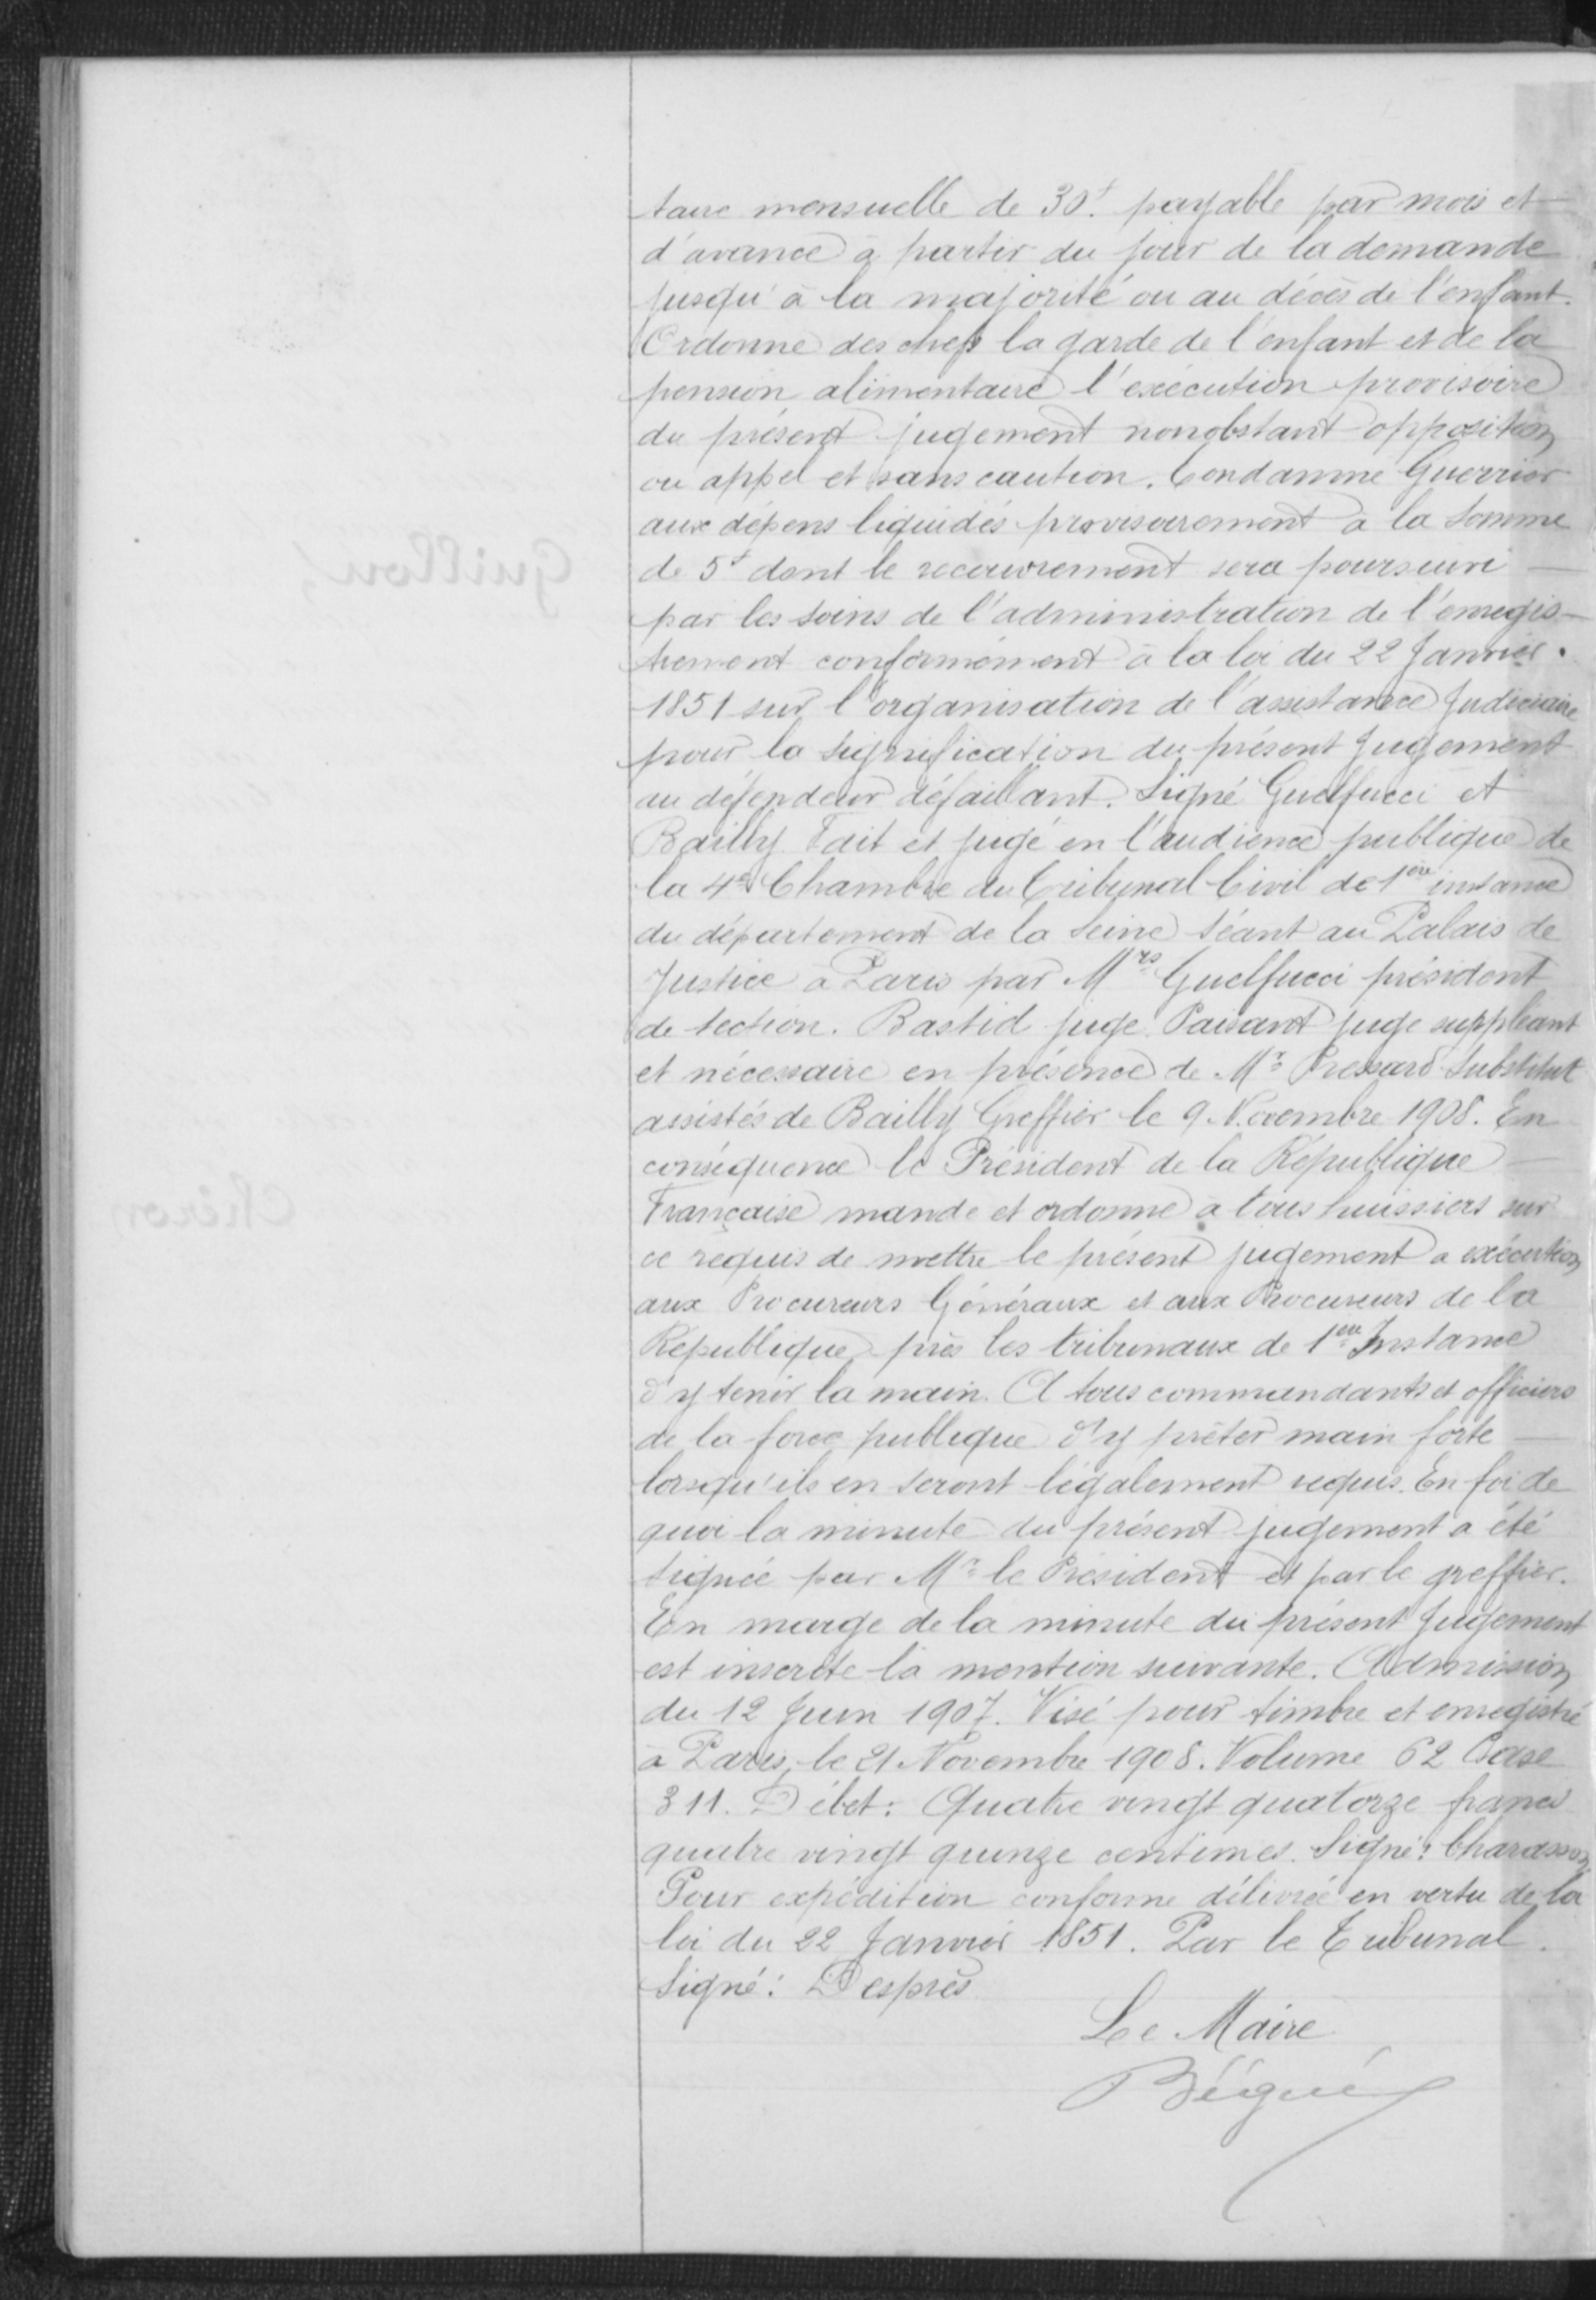taire mensuelle de 30t payable par mois et
d'avant à partir du jour de la demande
jusqu'à la majorité ou au décès de l'enfant.
Ordonne des chefs la garde de l'enfant et de la
pension alimentaire l'exécution provisoire
du présent jugement nonobstant opposition,
ou appel et sans caution. condamne Guerrier
aux dépens liquidés provisoirement à la Somme
de 5f dont le recouvrement sera poursuivi
par les soins de l'administration de l'enregis-
trement conformément à la loi du 22 Janvier
1851 sur l'organisation de l'assistance Judiciaire
pour la signification du présent Jugement
au defendeur défaillant. Signé Guelfucci et
Bailly. Fait et jugé en l'audience publique de
la 4° Chambre du Tribunal Civil de 1ere instance
du département de la Seine Séant au Palais de
Justice à Paris par Mre Guelfucci président
de section. Bastid Juge Pauvant juge suppléant
et nécessaire en présence de Me Preysard Substituant
assistés de Bailly Greffier le 9 Novembre 1909. En
conséquence el Président de la République
Française mande et ordonne à tous huissiers sur
ce requis de mettre le présent jugement a exécution
aux Procureur Généraux et aux Procureurs de la
République près les tribunaux de 1ere Instance
d'y tenir la main. Et tous commandants et officier
de la force publique s'y prêter main forte
lorsqu'ils en seront légalement requis. En foi de
quoi la minorité du présent jugement a été
signée par Mr le Président et par le greffier
En marge de la minute du présent Jugement
est inserte la mention suivante. Admission
du 12 Juin 1907. Visé pour timbre et enregistré
à Paris le 21 Novembre 1908. Volume 62 Cas
311. Débat: Quatre vingt quatorze franc
quatre vingt quinze centimes Signé: Charrassoz
Pour expédition conforme délivré en vertu de la
loi du 22 Janvier 1851. Par le Tribunal
Signé: Despres.
Le Maire
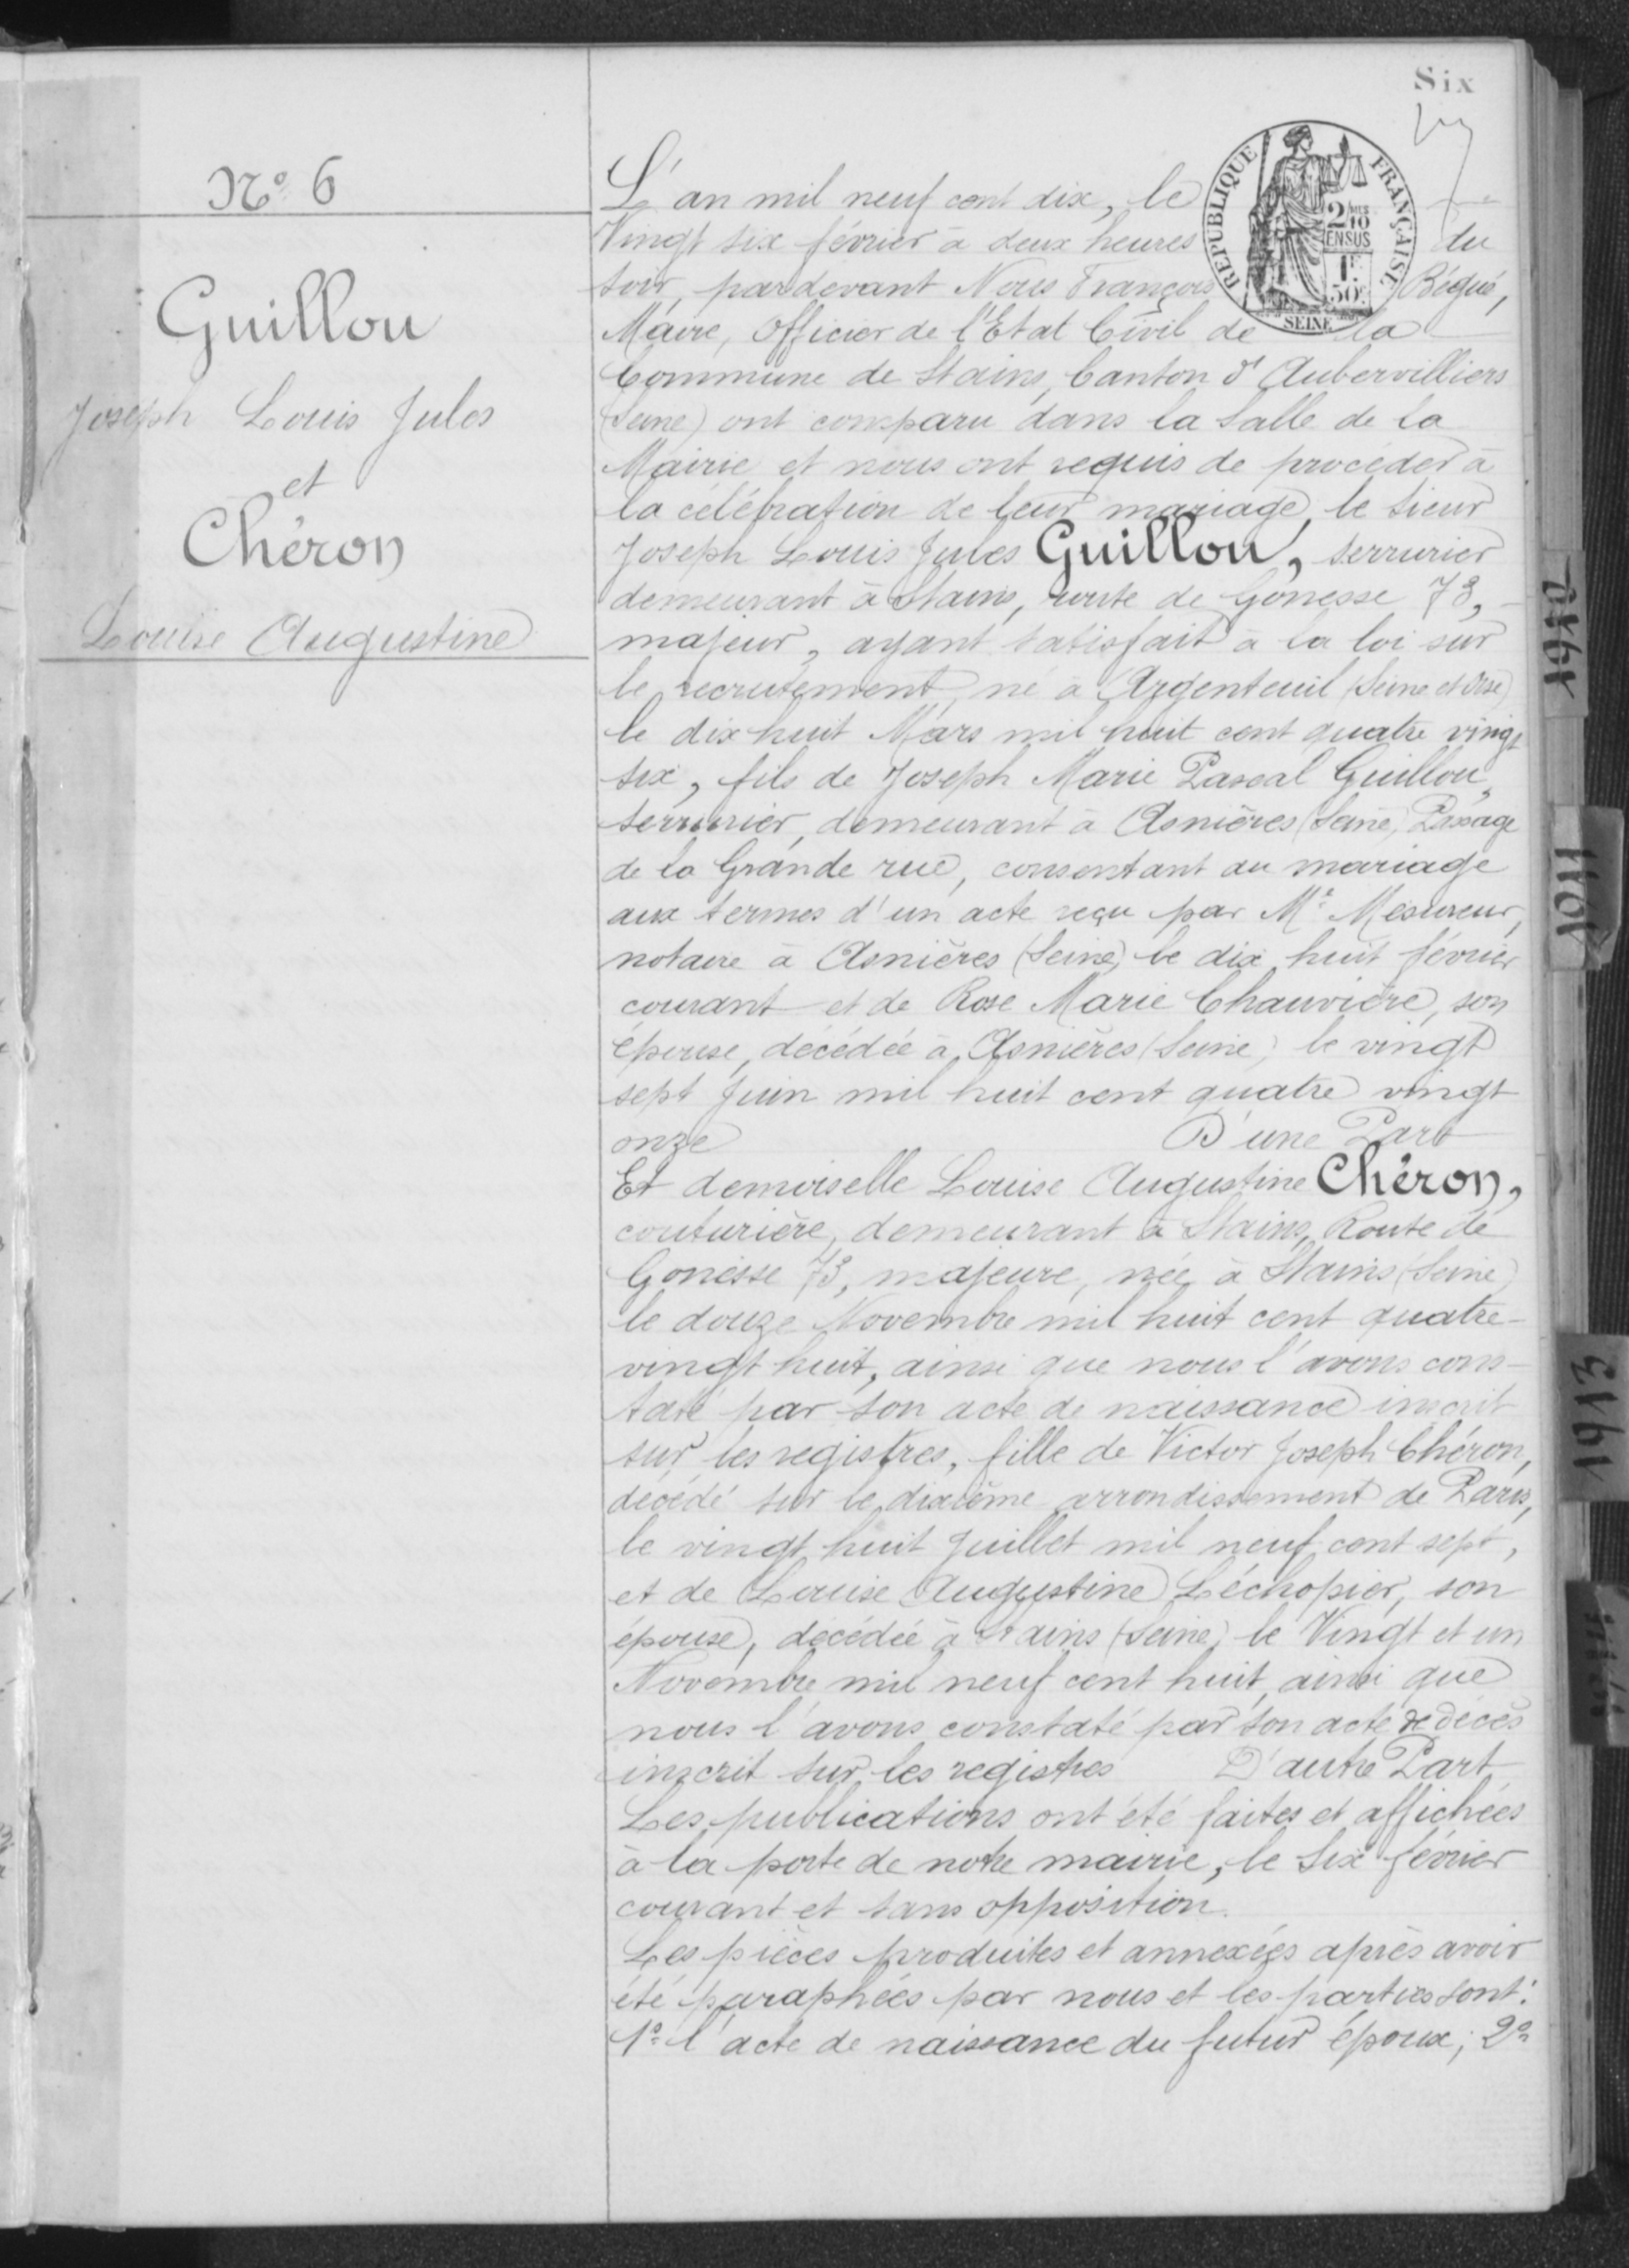N°6
Guillou
Joseph Louis Jules
et
Chéron
Louise Augustine
L'an mil neuf cent six, le
Vingt six février à deux heures du
soir, par devant Nous François Bégus,
Maire, Officier de l'Etat Civil de la
Commune de Stains, Canton d'Aubervillies
(Seine) ont comparu dans la Salle de la
mairie et nous ont requis de procéder à
la célébration de leur mariage le Sieur
Joseph Louis Jules Guillou, serrurier
demeurant à Stains, route de Gonesse, 73,
majeur, ayant satisfait à la loi sur
le recrutement, né à Argenteuil (Seine et Oise)
le dix huit Mars mil huit cent quatre vingt
six, fils de Joseph Marie Pasoal Guillou,
serrurier, demeurnt à Asnières (Seine) Laxage
de la Grande rue, consentant au mariage
aux termes d'un acte reçu par Me Mesureur,
Notaire à Asnières (Seine) le dix huit février
courant et de Rose Marie Chauvrière, son
épouse, décédée à Asnières (Seine) le vingt
sept juin mil huit cent quatre vingt
onze. D'une part
Et demoiselle Louis Augustine Chéron,
couturière, demeurant à Stains, Route de
Gonesse 73, majeure, née à Stains (Seine)
le douze Novembre mil huit cent quatre
vingt huit, ainsi que nous l'avons cons-
taté, par son acte de naissance inscrit
sur les registres, fille de Victor Joseph Chéron
décédé sur le dixième arrondissement de Paris
le vingt huit Juillet mil neuf cent sept
et de Louise Augustine Léchopier, son
épouse, décédée à Stains (Seine) le Vingt et un
Novembre mil neuf cent huit, ainsi que
nous l'avons constaté par son acte de décès
inscrit sur les registres D'autre Pars
Les publications ont été faites et affichées
à la porte de notre mairie le Six février
courant et sans opposition.
Les pièces produites et annexées après avoir
été paraphées par nous et les parties dont
1° l'acte de naissance du futur époux; 2°